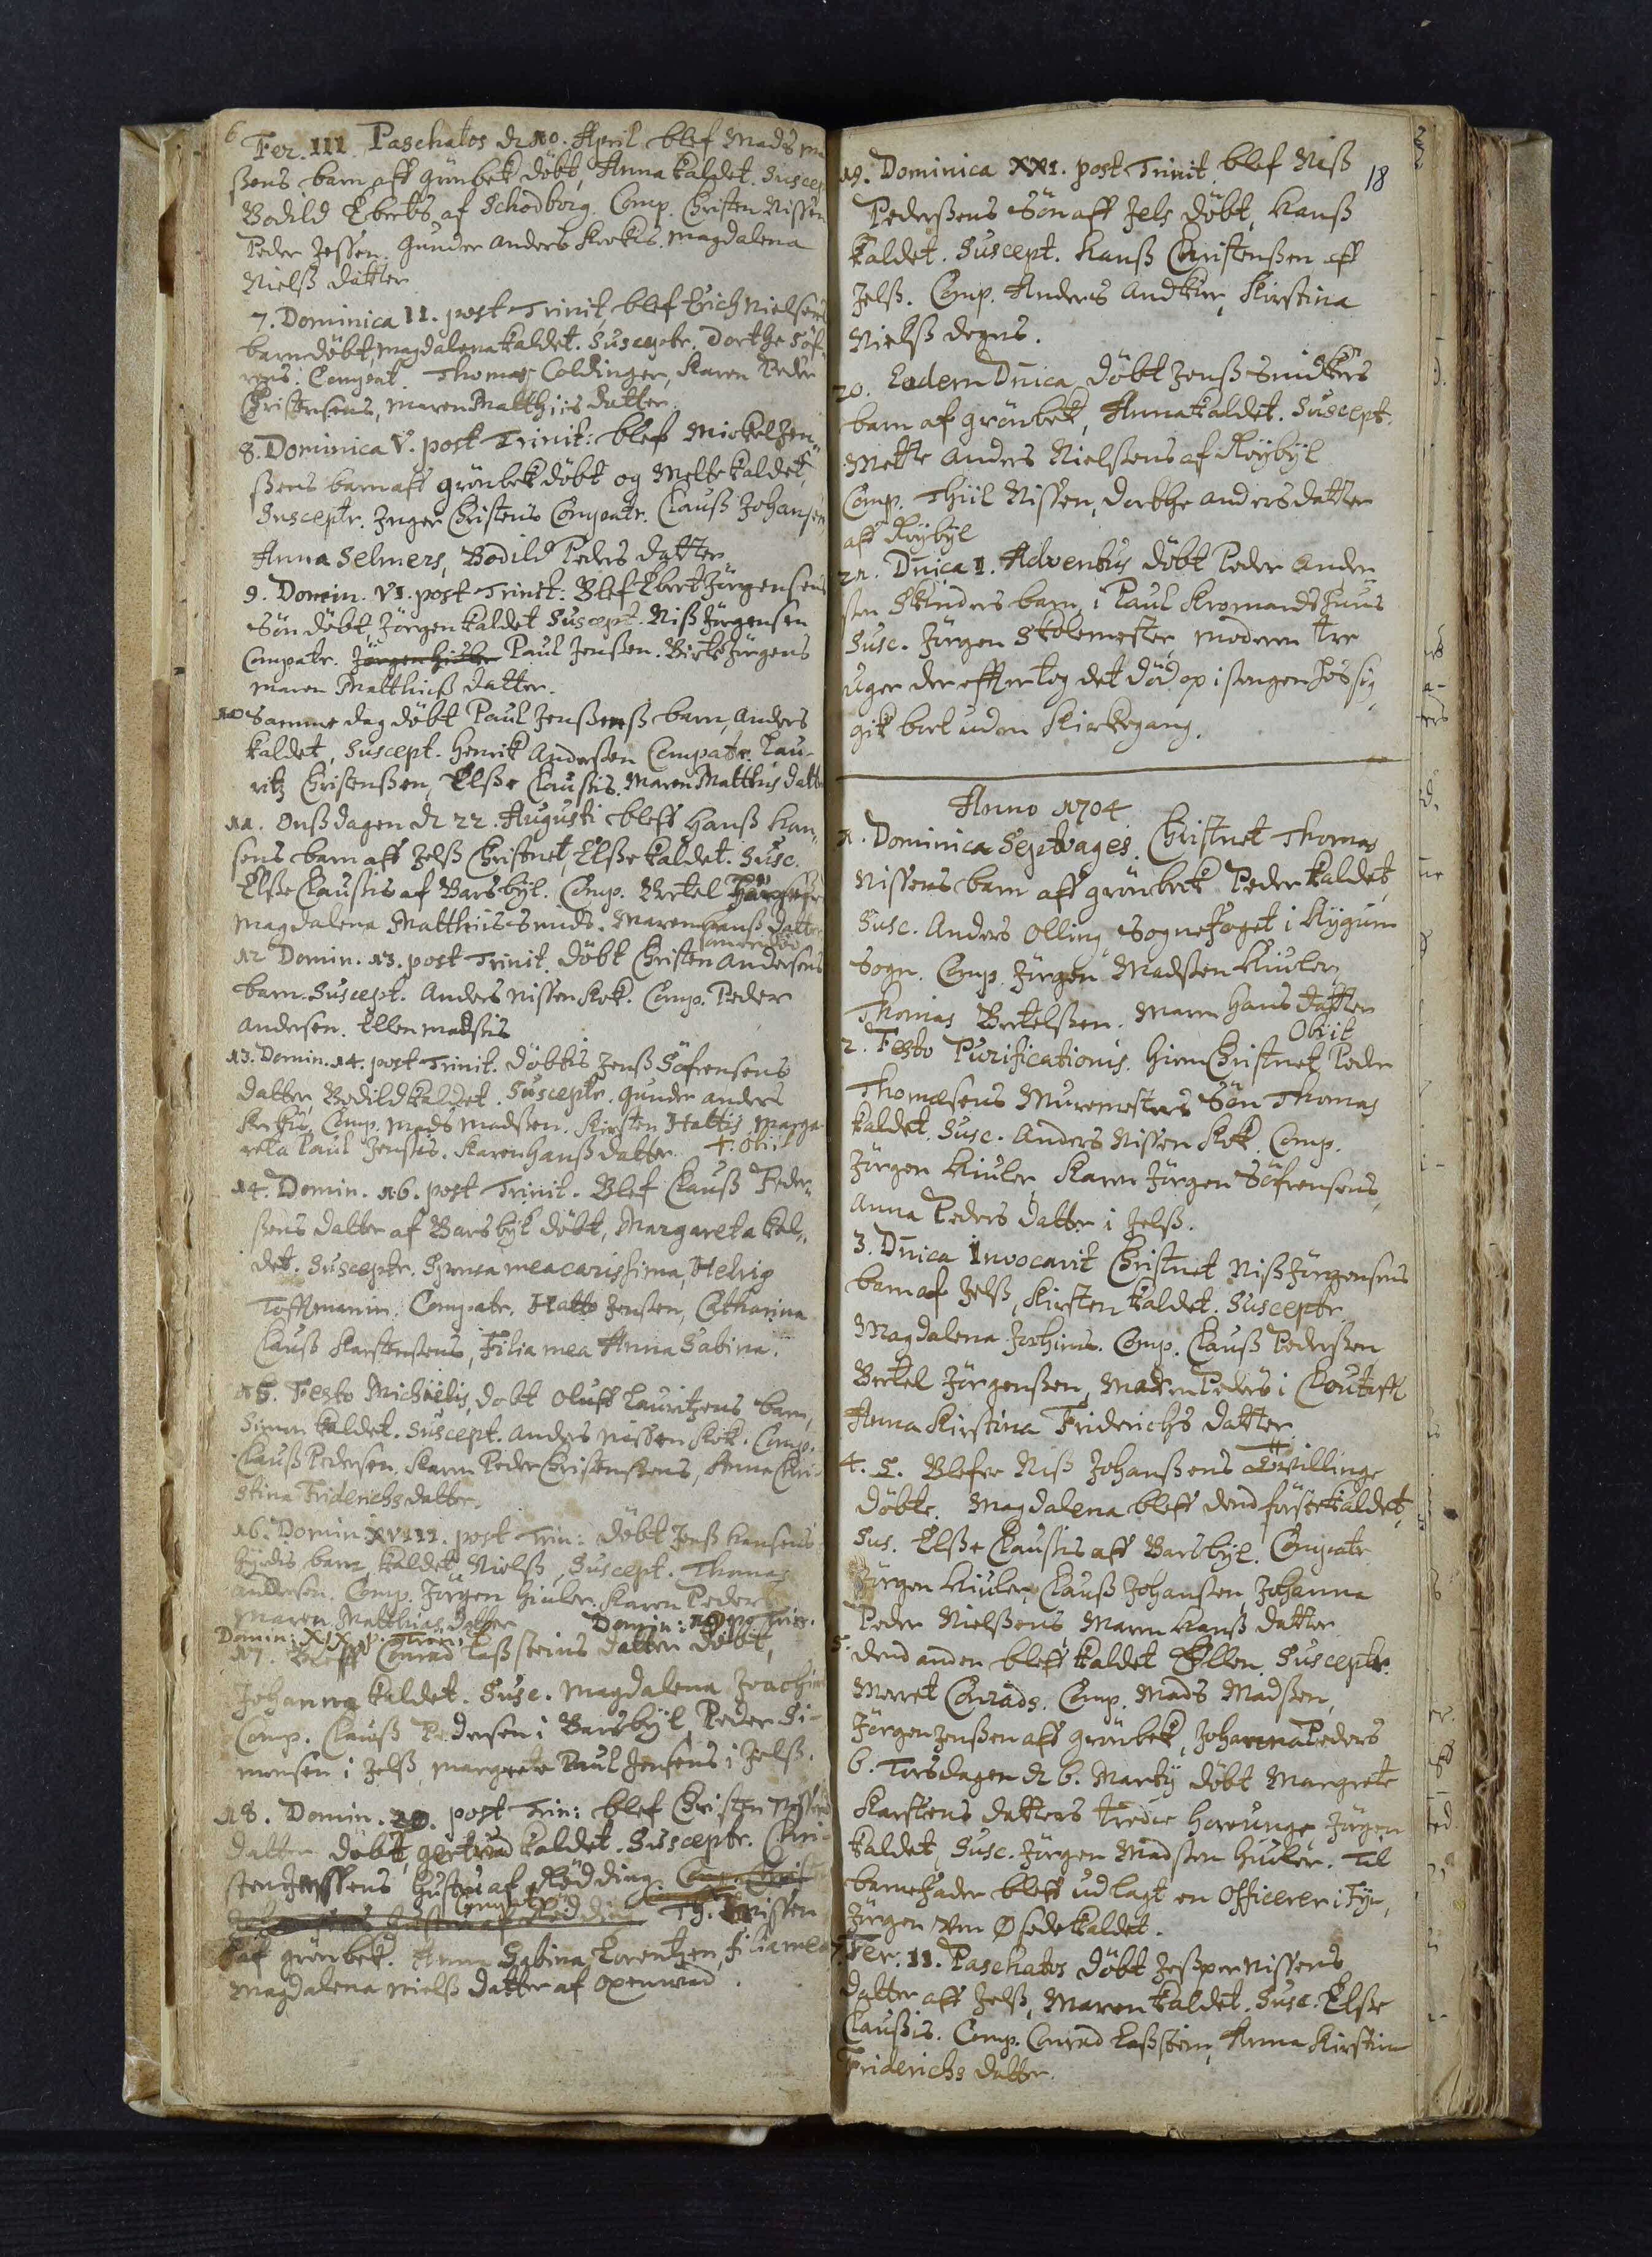6 Fer. III Pashatos d 10. April blef Mads Ma 
ssens barn aff Grønbek døbt, Anna kaldet. Suscep 
Bodild Ebertis af Schodborg. Comp. Christen Nissen
Peder Jessen. Gunder Anders Kockis. Magdalena
Nielss datter
7. Dominica II. post Trinit blef Erich Nielsens
barn døbt, Magdalena kaldet. Susceptr. Dorthe Søf¬
rens Compret. Thomas Coldinger, Karen
Christensens, Maren Matthiis Datter
8. Dominica V. post Trinit. blef Mickel Jen¬
sens barn aff Grønbek døbt og Mette kaldet
Susceptr. Inger Christens Compatr. Claus Johansen,
Anna Selmers, Bodild Peders datter
9. Domin. VI. post Trinit: Blef Ebert Jørgensens
Søn døbt, Jørgen kaldet. Suscept. Niss Jørgensen
Compatr. Jørgen Hiuler Paul Jensen. Birte Jørgens
Maren Matthiess datter.
10. Samme dag døbt Paul Jenssenss barn, Anders
kaldet, suscept. Henrik Andersen. Compatr. Lau¬
ritz Christenssen, Elsse Claussis, Maren Matthies datt 
11. Onsdagen d 22. Augusti bleff Hans Han¬
sens barn aff Jelss Christnet, Elsse kaldet. Susc.
Elsse Claussis af Barsbyl. Comp. Bertel
Magdalena Matthies Smids. Maren Hanss datter
##
12. Domin. 13. post Trinit. døbt Christen Andersens
barn. Suscept. Anders Nissen Kok. Comp. Peder
Andersen. Ellen Madsis
13. Domin. 14. post Trinit. døbtis Jenss Søfrensens
Datter , Bodild kaldet. Susceptr. Gunder Anders
Kockis. Comp. Mads Madsen. Kirsten Hattis Marga¬
reta Paul Jensis. Karen Hanss datter
Obiit
14. Domin. 16. post Trinit. Blef Claus Peder¬
ssens datter af Barsbyl døbt, Margareta kal—
det. Susceptr. ##a meacarissima, Helvig
Tofftmann. Compatr. Hatto Jensen, Catharina
Clauss Karstensens, Filia mea Anna Sabina.
15. Festo Michaelis døbt Oluff Lauritsens barn,
Simen kaldet. Suscept. Anders Nissen Kok. Comp.
Clauss Pedersen. Karen Peder Christensens, Anna Chri
stina Friderichs datter.
16. Domin. XVIII. post Trin. døbt Jens Hansen
Hyrdis barn, kaldet Nielss, Suscept. Thomas
Andersen. Comp. Jørgen Hiuler. Karen Peders
Maren Matthias datter
Domin: ## p## Trin.
Domin XIX p. Trin
17. blef Conrad Lassteins datter døbt,
Johanna kaldet. Susc. Magdalena Joachims
Comp. Clauss Pedersen i Barsbyl, Peder Si¬
mensen i Jelss, Margrete Paul Jensens i Jelss.
18. Domin. 20. post Trin: blef Christen Nissens
datter døbt, Gertrud kaldet. Susceptr. Chri¬
sten J##ens Hustru af Rødding.
Comp
T##. ##sen
af Grønbek. Anna Sabina Lorentzen, fi##me##
Magdalena Nielss datter af Oxenvad
18
19. Dominica XXI. Post Trinit blef Niss
Pedersens Søn aff Jels døbt, Hanss
kaldet. Suscept. Hans Christensen aff
Jelss. Comp. Anders Andkier, Kirstina
Nielss degns.
20. Eadem Dnica døbt Jenss Snidkers
barn af Grønbek, Anna kaldet. Suscept.
Mette Anders Nielsens af Roybyl.
Comp. Thiil Nissen, Dorthe Andersdatter
aff Roybyl
21. Dnica I. Adventus døbt Peder Ander¬
sen Skinders barn i Paul Kromands huus
Susc. Jørgen Skolemester, moderen tre
uger der effter tog det død op i sengen hos sig
gik bort uden Kirkegang.
Anno 1704
1. Dominica Septvages. Christnet Thomas
Nissens barn aff Grønbeck, Peder kaldet
Susc. Anders Olling Sognefoget i Hygum
Sogn. Comp. Jørgen Madsen Hiuler
Thomas Bertelsen. Maren Hans datter
Obiit
2. Festo Purificationis. Hiem Christnet Peder
Thomæsens Muremesters Søn Thomas
kaldet. Susc. Anders Nissen Kok. Comp.
Jørgen Hiuler, Karen Jørgen Søfrensens
Anna Peders datter i Jelss.
3. Dnica Invocavit Christnet Niss Jørgensens
barn af Jelss, Kirsten kaldet. Susceptr.
Magdalena Jochims. Comp. Clauss Pedersen
Bertel Jørgenssen, Ma## Peders i Cloutofft
Anna Kirstina Friderichs datter.
4. 5. Blefve Niss Johansens Tvillinge
døbte. Magdalena bleff dend første kaldet, sus.
Elsse Clausis aff Barsbyl. Compatr.
Jørgen Hiuler, Clauss Johansen, Johanne
Peder Nielssens, Maren Hanss datter
5. Dend anden bleff kaldet Ellen. Susceptr.
Merret Conrads. Comp. Mads Madsen, 
Jørgen Jenssen aff Grønbek, Johanna Peders
6. Torsdagen d 6. Martij døbt Margrete
Karstens datters tredie horeunge, Jørgen
kaldet. Susc. Jørgen Madsen Hiuler. Til
barnefader bleff udlagt en Officerer i Fyn, 
Jørgen von Øsede kaldet.
7. Fer. II. Paschatos døbt Jessper Nissens
datter aff Jelss, Maren kaldet. Susc. Elsse
Claussis. Comp. Conrad Lasstein, Anna Kirstine
Friderichs datter.